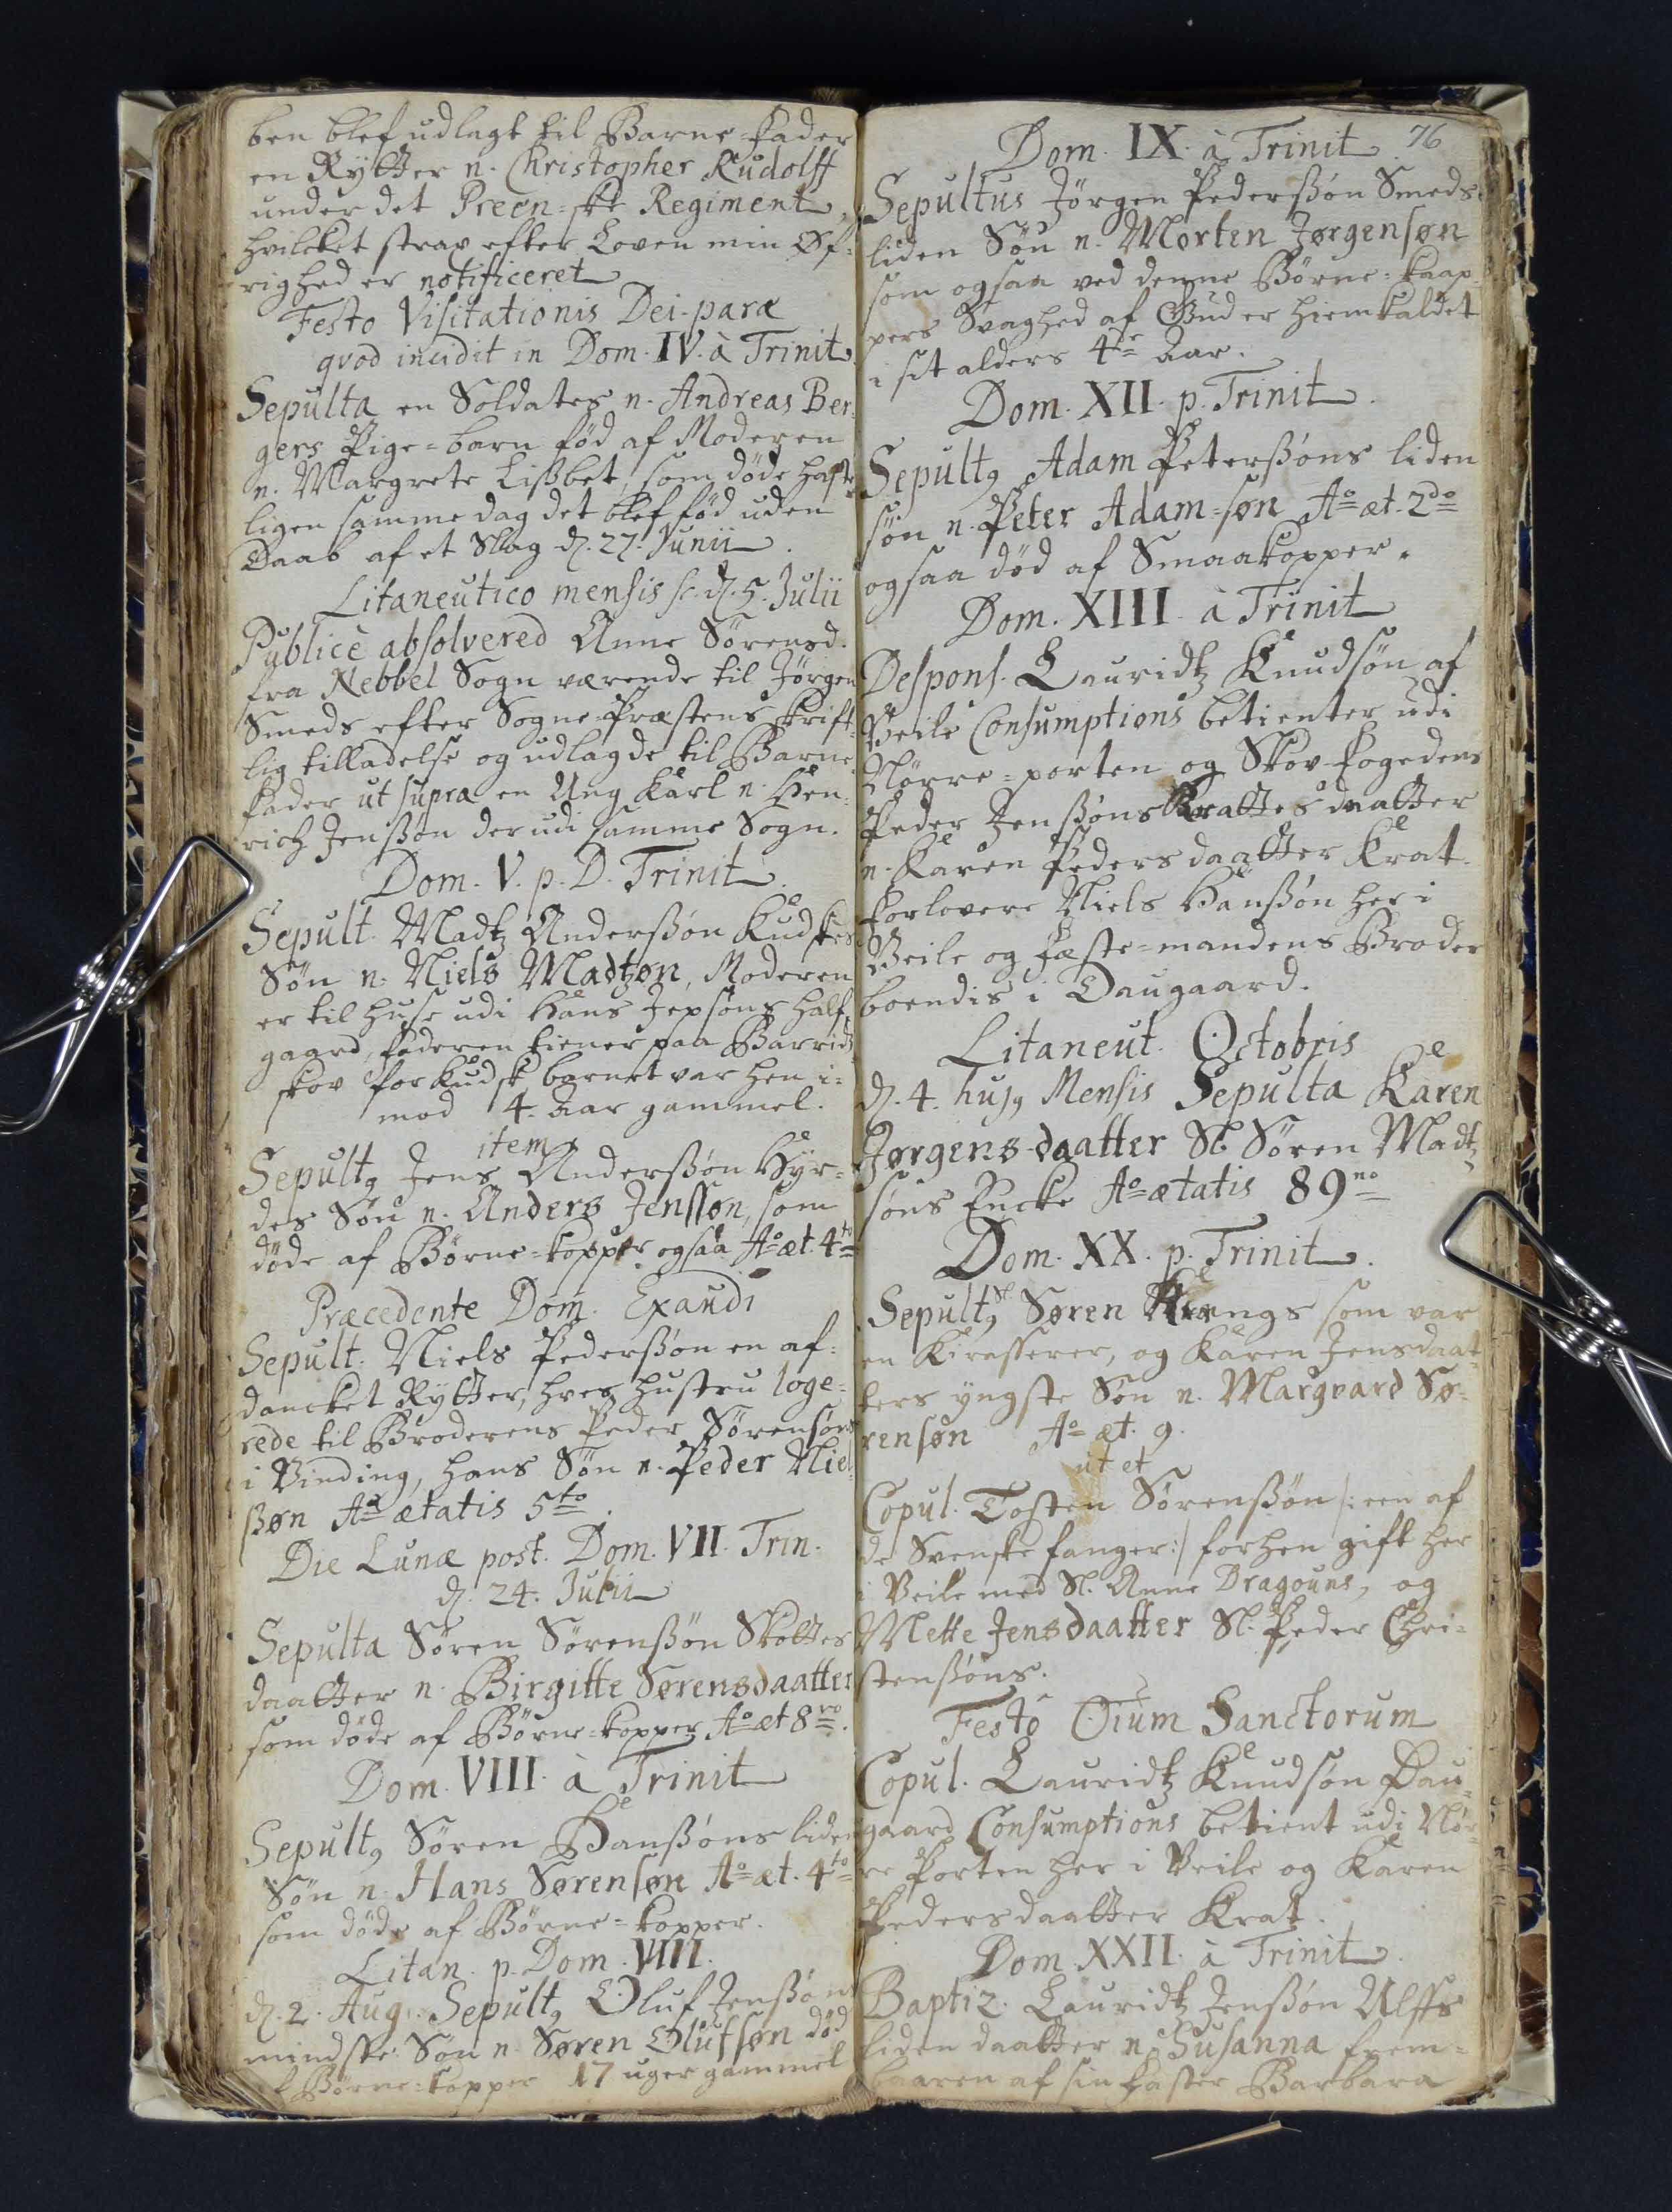ben blef udlagt til Barne=Fader
en Rytter n. Christopher Rudolff 
under det Preen=ske Regiment
hvilket strax efter Loven min Øf¬
righed er notificeret
Festo Visitationis Dei - paræ
qvod incidit in Dom. IV. a Trinit.
Sepulta en Soldates n. Andreas Ber¬
gers Pige=barn, fød af Moderen
n. Margrete Lissbet, som døde haste
ligen samme dag det blef fød uden
Daab af et Slag d. 27. Junii
Litaneutico mensis sc. d. 5 Julii
Publice absolvered Anne Sørensd.
fra Nebbel Sogn værende til Jørgen
Smeds efter Sogne=Præstens skrift¬
lig tilladelse og udlagde til Barne
Fader ut supra en Ung Karl n. Hen¬
rich Jenssøn der udi samme Sogn.
Dom. V. p. D. Trinit
Sepult. Madtz Anderssøn Kudskes
Søn n. Niels Madtzøn, Moderen
er til huse udi Hans Jepsøns half¬
gaard, faderen tiener paa Barridz¬
skov for Kudsk barnet var hen i¬
mod 4. Aar gammel.
item
Sepultq Jens Anderssøn Hyr¬
des Søn n. Anders Jenssøn, som
døde af Børne=kopper ogsaa Ao æt. 4to
Præcedente Dom. Exaudi
Sepult. Niels Pederssøn en af¬
dancket Rytter, hves hustru loge¬
rede til Broderens Peder Sørensøns
i Vinding, hans Søn n. Peder Niel¬
ssøn Ao ætatis 5to
Die Lunæ post. Dom. VII. Trin.
d. 24. Julii
Sepulta Søren Sørenssøn Skottes
daatter n. Birgitte Sørensdaatter
som døde af Børne=kopper Ao æt 8##
Dom. VIII. a Trinit
Sepultq Søren Hanssøns liden
Søn n. Hans Sørensøn Ao æt. 4to
som døde af Børne=kopper.
Litan p. Dom. VIII.
d. 2. Aug. Sepultq Oluf Jenssøns
mindste Søn n. Søren Olufsøn, død
f Børne=kopper 17 uger gammel
76
Dom. IX. a Trinit
Sepultus Jørgen Pederssøn Smeds
liden Søn n. Morten Jørgensøn
som ogsaa ved denne Børne=koop¬
pers Svaghed af Gud er hiemkaldet 
i sit alders 4de Aar.
Dom. XII. p. Trinit
Sepultq Adam Peterssøns liden
søn n. Peter Adam=søn Ao æt. 2do
ogsaa død af Smaakopper.
Dom. XIII. a Trinit
Despons. Lauridtz Knudsøn af
Veile Consumptions betienter udi
Nørre=porten og Skov-fogedens
Peder Jenssøns Krattes daatter
n. Karen Pedersdaatter Krat
Forlovere Niels Hanssøn her i
Veile og Fæste=mandens Broder 
boendis i Daugaard.
Litaneut. Octobris.
d. 4. hujq Mensis Sepulta Karen
Jørgens-daatter Sl. Søren Madtz
søns Encke Ao ætatis 89no
Dom. XX. p. Trinit
Sl.
Sepultq Søren Krengs som var
en Kirasserer, og Karen Jensdaat¬
ters yngste Søn n. Marqvard Sø¬
rensøn Ao æt. 9.
ut et
Copul. Tosten Sørenssøn /: een af
de Svenske fanger :/ forhen gift her
i Veile med Sl. Anne Dragouns, og
Mette Jensdaatter Sl. Peder Chri¬
stensøns
Festo Oium Sanctorum
Copul. Lauridtz Knudsøn Dau¬
gaard Consumptions betient udi Nør¬
re Porten her i Veile og Karen
Pedersdaatter Krat.
Dom. XXII. a Trinit
Baptiz. Lauridtz Jenssøn Ulffs
liden daatter n. Susanna, frem¬
baaren af sin faster Barbara 
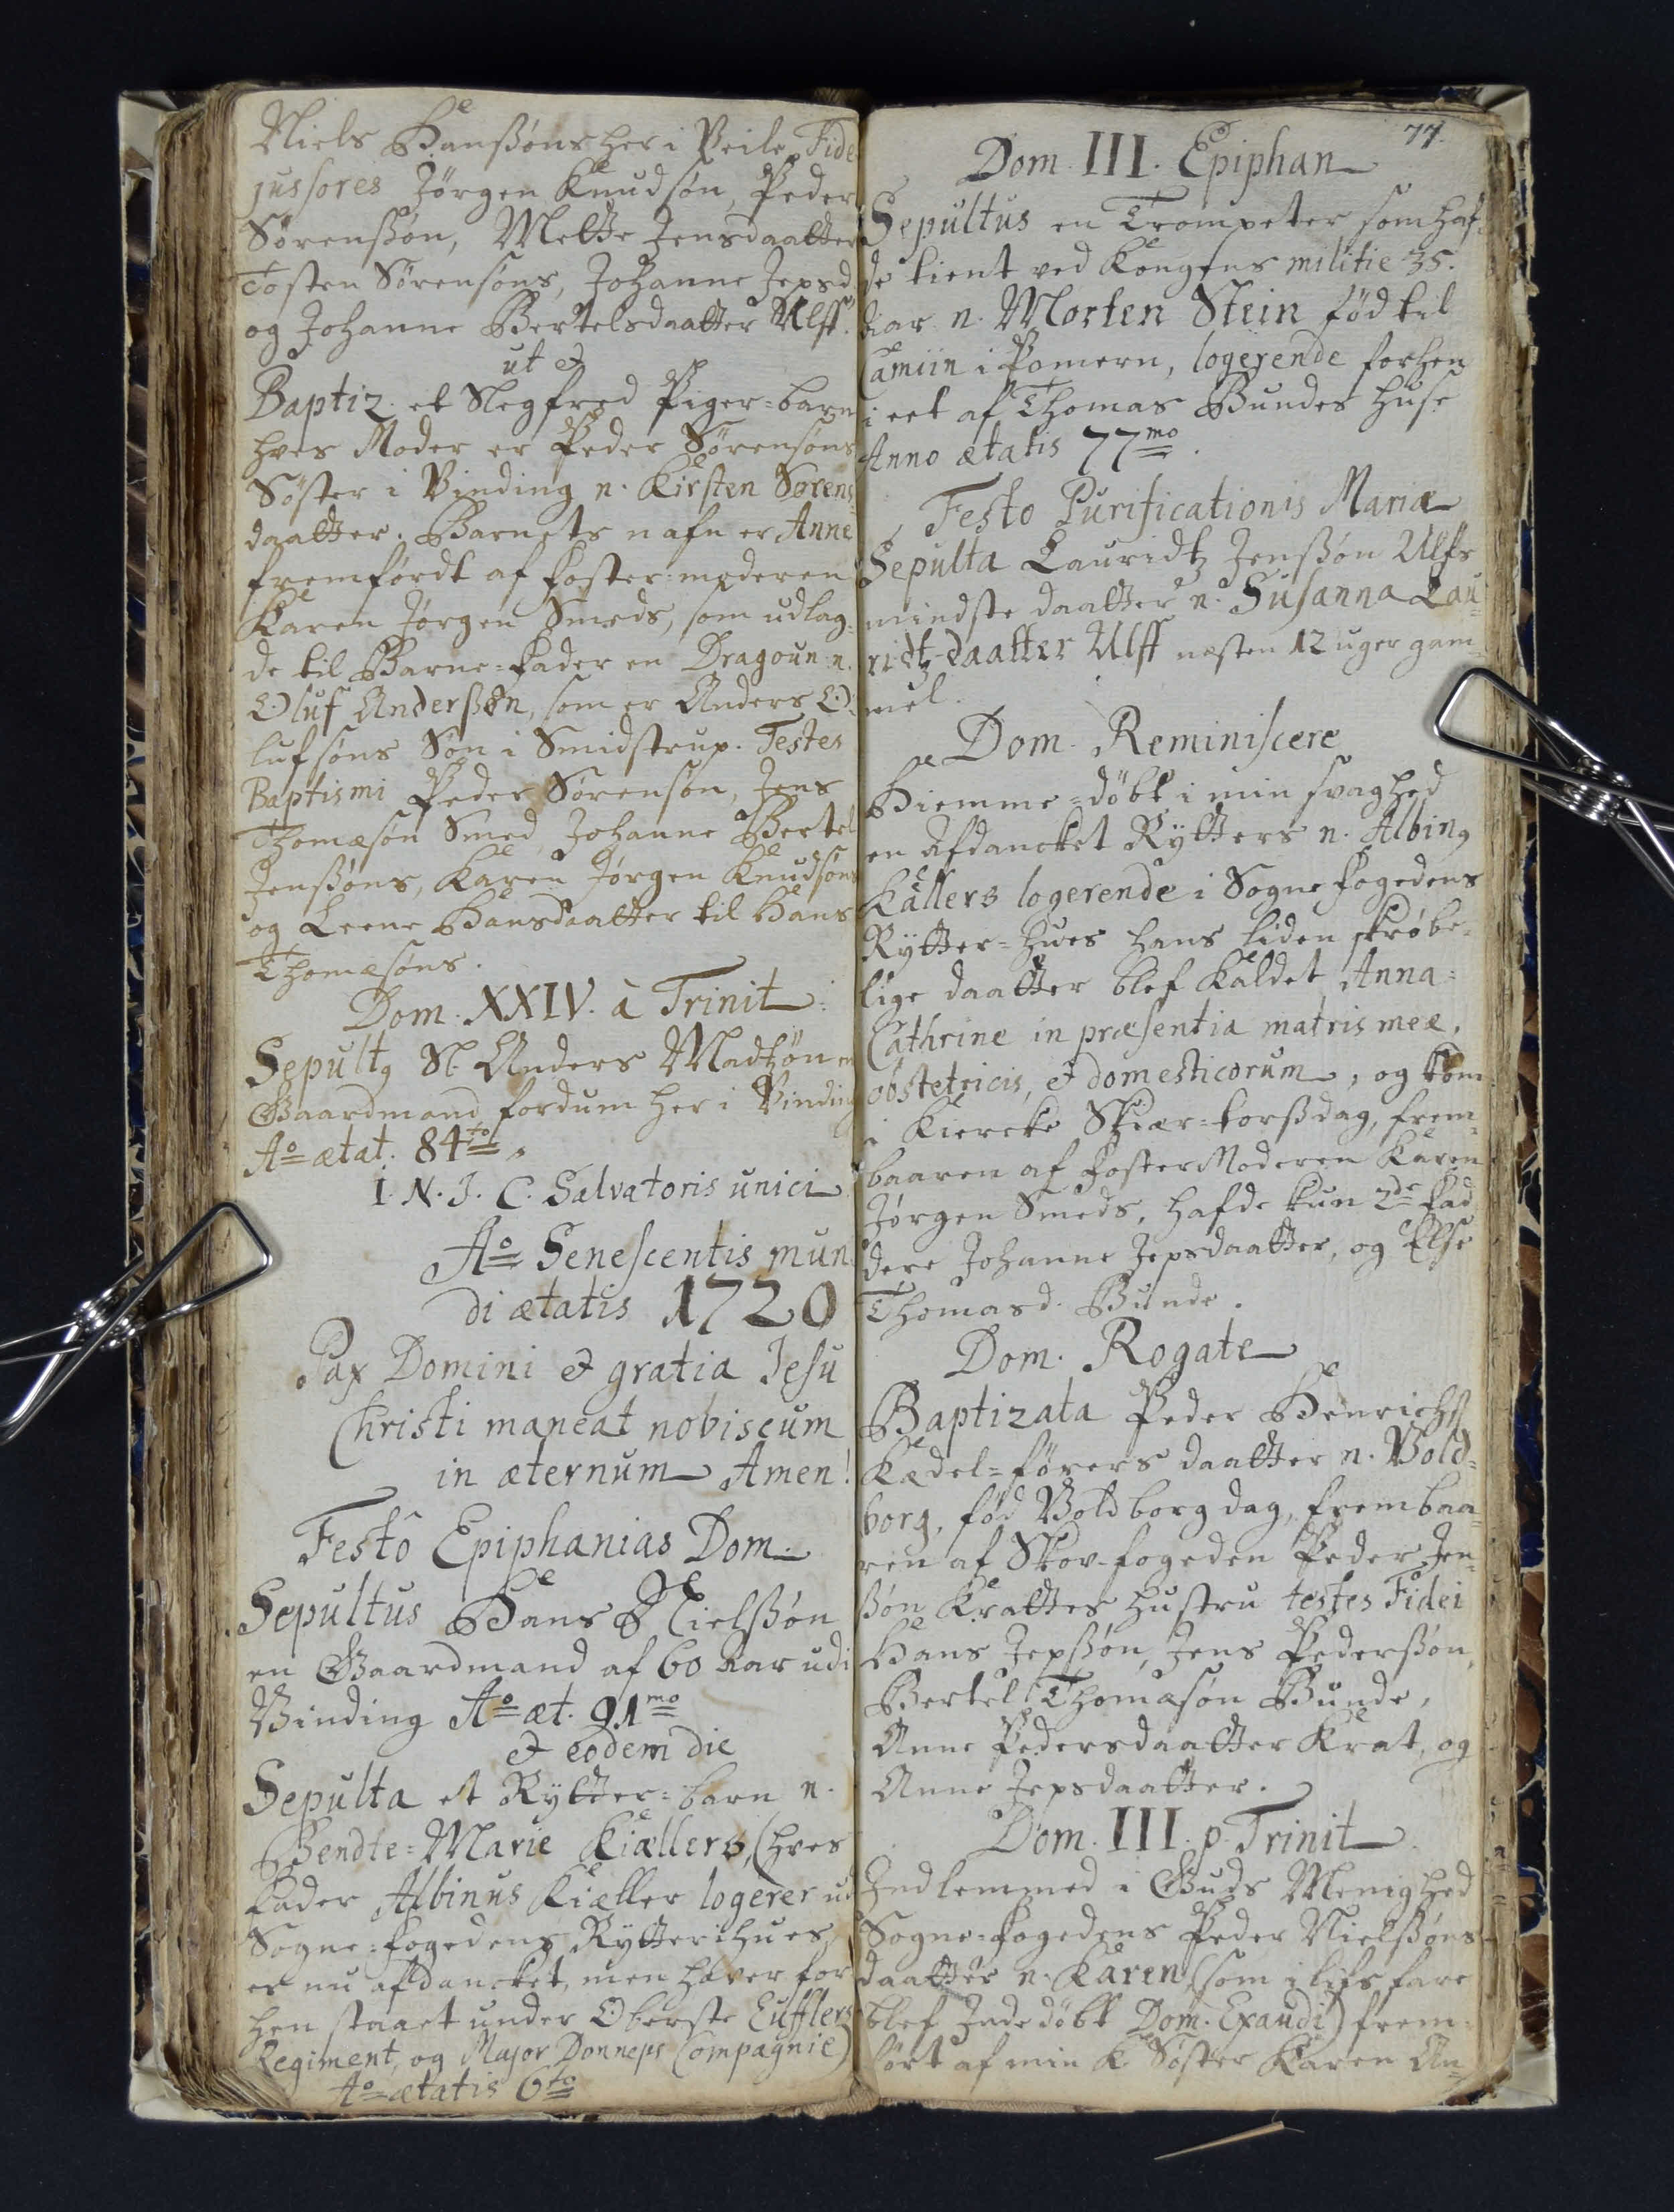Niels Hanssøns her i Veile Fide
jussores Jørgen Knudsøn, Peder
Sørenssøn, Mette Jensdaatter
Tosten Sørensøns, Johanne Jepsd.
og Johanne Bertelsdaatter Ulff.
ut et
Baptiz. et Slegfred Pige=barn
hves Moder er Peder Sørensøns
Søster i Vinding n. Kirsten Sørens¬
daatter. Barnets nafn er Anne
fremførdt af Foster=moderen
Karen Jørgen Smeds, som udlag¬
de til Barne=fader en Dragoun n.
Oluf Anderssøn, som er Anders O¬
lufsøns Søn i Smidstrup. Tesates
Baptismi Peder Sørensøn, Jens
Thomæsøn Smed, Johanne Bertel
Jenssøns, Karen Jørgen Knudsøns
og Leene Hansdaatter til Hans
Thomæsøns
Dom. XXIV. a Trinit
Sepultq Sl. Anders Madtzøn en
Gaardmand fordum her i Vinding
Ao ætat. 84to.
I. N. J. C. Salvatoris unici
Ao Senescentis mun¬
di ætatis 1720
Pax Domini et gratia Jesu
Christi maneat nobiscum
in æternum Amen!
Festo Epiphanias Dom.
Sepultus Hans Nielssøn
en Gaardmand af 60 Aar udi
Vinding Ao æt. 91mo
et eodem die 
Sepulta et Rytter=barn n.
Bendte=Marie Kiællers (hves
Fader Albinus Kiæller logere ud 
Sogne=fogedens Rytter i hues,
er nu afdancket, men haver for
hen staaet under Oberste Eufflers
Regiment, og Major Donneps Compagnie)
Ao ætatis 6to
77.
Dom. III Epiphan.
Sepultus en Trompeter som haf¬
de tient ved Kongens militie 35.
Aar n. Morten Stein, fød til 
Camiin i Pomern, logerende forhen 
i eet af Thomas Bundes huse 
Anno ætatis 77mo.
Festo Purificationis Maria
Sepulta Lauridtz Jenssøn Ulfs
mindste daatter n. Susanna Lau¬
ridtz-daatter Ulff næsten 12 uger gam¬
mel.
Dom. Reminiscere
Hiemme=døbt i min svaghed
en Afdancket Rytter n. Albinq
Kallers logerende i Sogne Fogedens
Rytter=hues hans liden skrøbe¬
lige daatter blef kaldet Anna
Cathrine in præsentia matris meæ,
obstetricis, et domesticorum, og kom
i Kiercke Skiær=torssdag, frem¬
baaren af Foster Moderen Karen
Jørgen Smeds, hafde kun 2de fad¬
dere Johanne Jepsdaatter, og Else
Thomasd. Bunde
Dom. Rogate
Baptizata Peder Henrichs
Kædel=førers daatter n. Vold¬
borg, fød Voldborg dag, frembaa¬
ren af Skov=fogeden Peder Jen¬
ssøn Krattes hustru testes Fidei
Hans Jepssøn, Jens Pederssøn
Bertel Thomæsøn Bunde,
Anne Pedersdaatter Krat, og
Anne Jepsdaatter.
Dom. III. p. trinit
Indlemmed i Guds Menighed
Sogne=Fogedens Peder Nielssøns
daatter n. Karen (som i lifs fare
blef Inde døbt Dom. Exaudi) frem¬
ført af min K. Søster Karen An¬